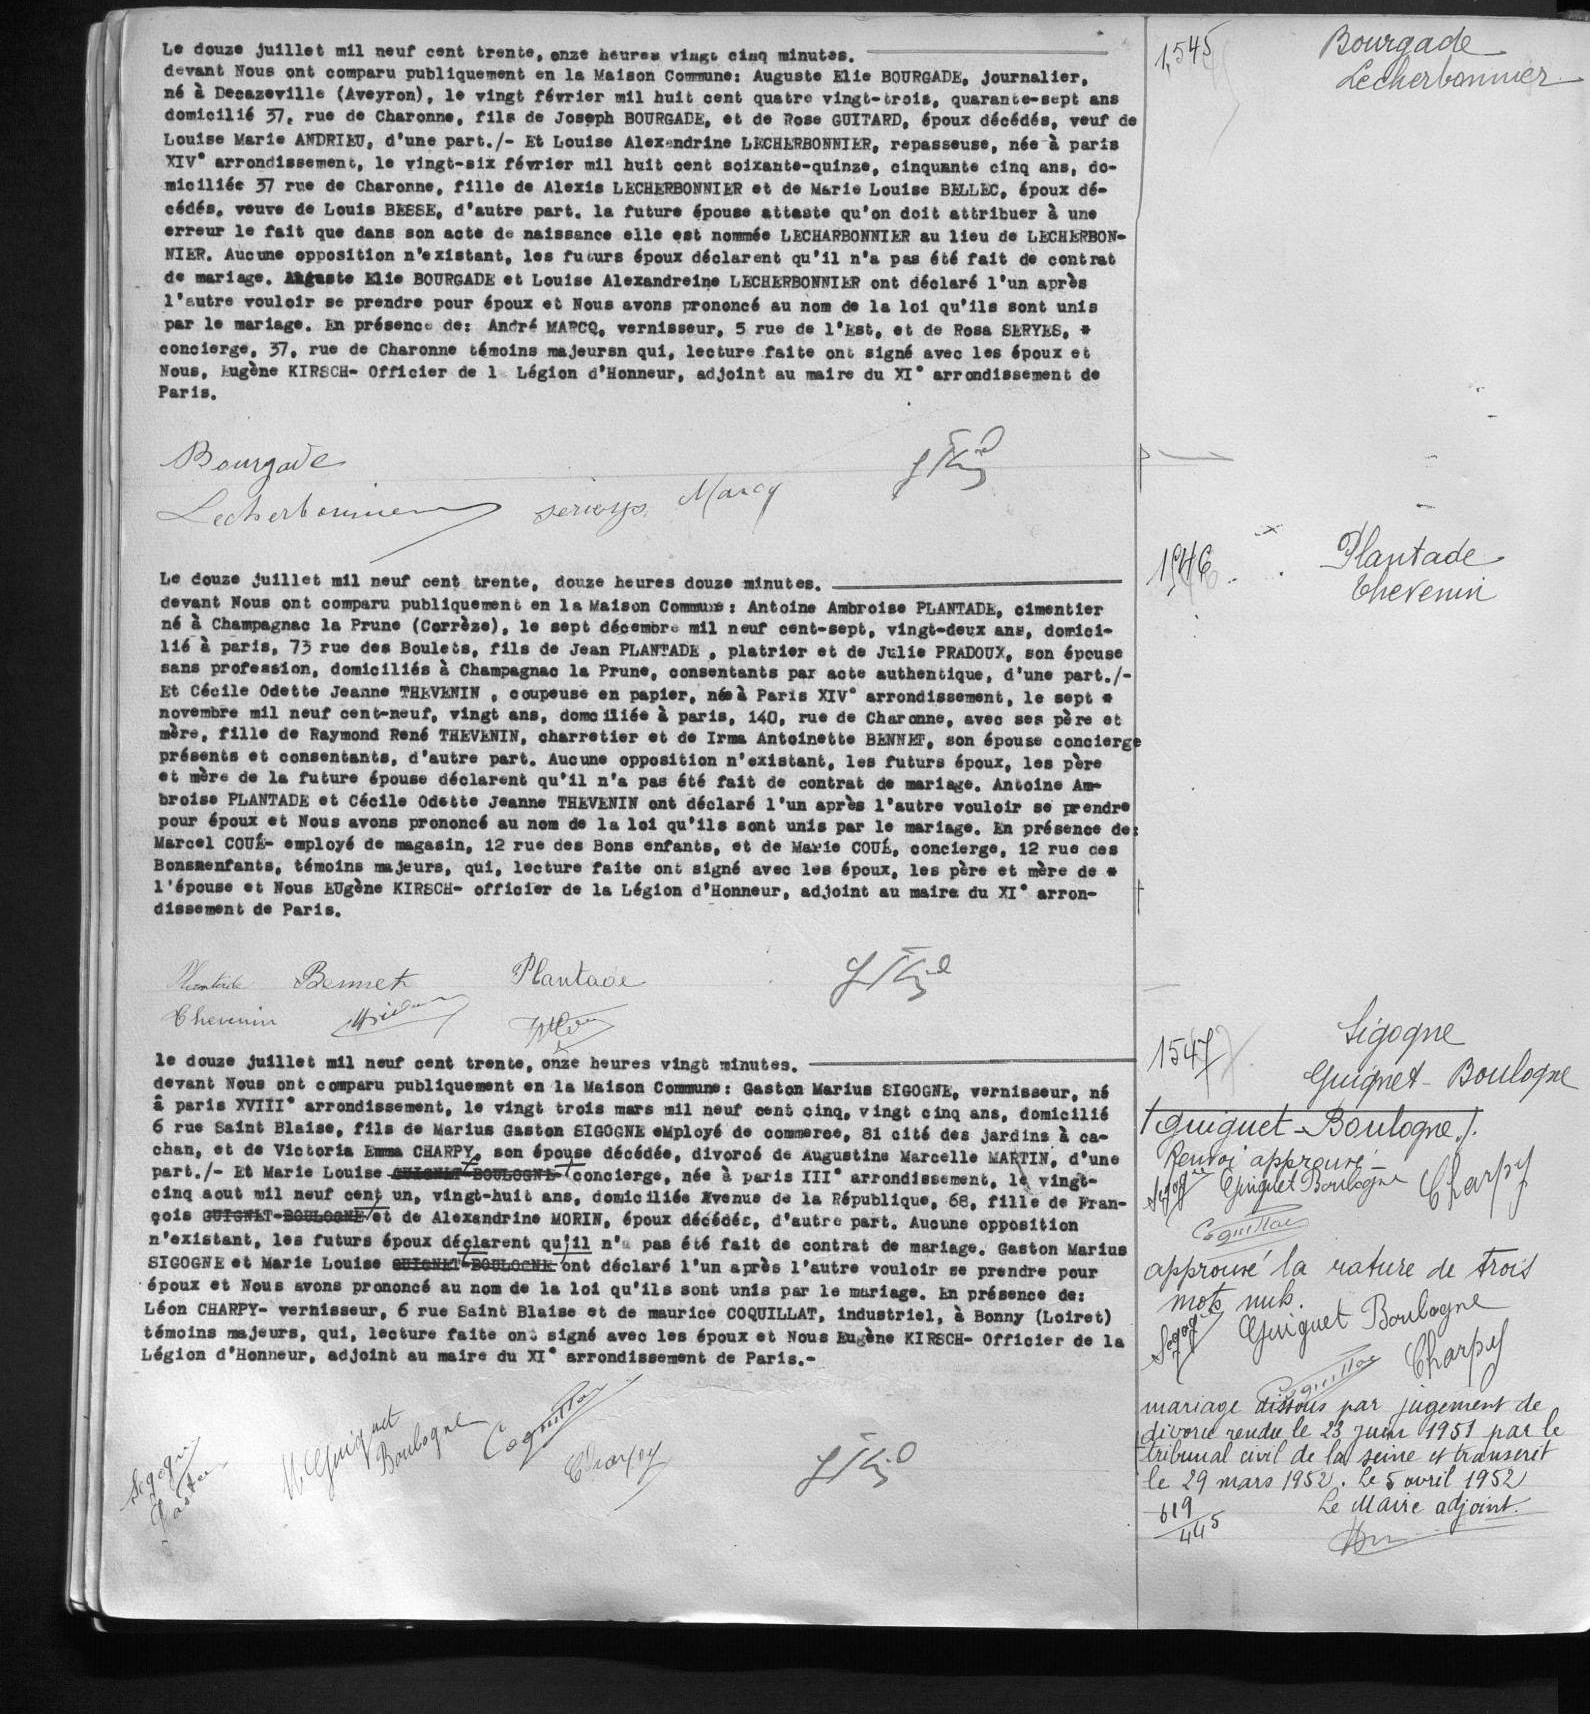Le douze juillet mil neuf cent trente, onze heures vingt minutes ,-
devant Nous ont comparu publiquement en la Maison Commune: Auguste Elie BOURGADE, journalier,
né à Decazeville (Aveyron), le vingt février mil huit cent quatre vingt-trois, quarante-sept ans
domicilié 37, rue de Charonne, fils de Joseph BOURGADE, et de Rose GUITARD, époux décédés, veuf de
Louise Marie ANDRIEU, d'une part ./-Et Louise Alexandrine LECHERBONNIER, repasseuse, née à paris
XIV ° arrondissement, le vingt-six février mil huit cent soixante-quinze, cinquante cinq ans, do-
miciliée 37 rue de Charonne, fille de Alexis LECHERBONNIER et de Marie Louise BELLEC, époux dé-
cédés, veuve de Louis BESSE, d'autre part. La future épouse atteste qu'on doit attribuer à une
erreur le fait que dans son acte de naissance elle est nommé LECHARBONNIER au lieu de LECHERBON-
NIER. Aucune opposition n'existant, les futurs époux déclarent qu'il n'a pas été fait decontrat
de mariage. Auguste Elie BOURGADE et Louise Alexandreine LECHERBONNIER ont déclaré l'un après
l'autre vouloir se prendre pour époux et Nous avons prononcé au nom de la loi qu'ils sont unis
par le mariage. En présence de: André MARCQ, vernisseur, 5 rue de l'Est, et de Rosa SEYRES, *
concierge, 37, rue de Charonne témoins majeursn qui, lecture faite ont signé avec les époux et
Nous, Eugène KIRSCH-Officier de l Légion d'Honneur, adjoint au maire du XI ° arrondissement de
Paris.
Le douze juillet mil neuf cent trente, douze heures douze minutes .-
devant Nous ont comparu publiquement en la Maison Commune: Antoine Ambroise PLANTADE, cimentier
né à Champagnac la Prune (Corrèze), le sept décembre mil neuf cent-sept, vingt-deux ans, domici-
lié à paris, 73 rue des Boulets, fils de Jean PLANTADE, platrier et de Julie PRADOUX, son épouse
sans profession, domiciliés à Champagnac la Prune, consentants par acte authentique, d'une part ./-
Et Cécile Odette Jeanne THEVENIN, coupeuse en papier, née à Paris XIV ° arrondissement, le sept*
novembre mil neuf cent-neuf, vingt ans, domiciliée à paris, 140, rue de Charonne, avec ses père et
mère, fille de Raymond René THEVENI, charretier et de Irma Antoinette BENNET, son épouse concierge
présents et consentants, d'autre part. Aucune opposition n'existant, les futurs époux, les père
et mère de la future épouse déclarent qu'il n'a pas été fait de contrat de mariage. Antoine Am-
broise PLANTADE et Cécile Odette Jeanne THEVENIN ont déclaré l'un après l'autre vouloir se prendre
pour époux et Nous avons prononcé au nom de la loi qu'ils sont unis par le mariage. En présence de:
Marcel COUE-employé de magasin, 12 rue de Bons enfants, et de Marie COUE, concierge, 12 rue ces
Bonsenfants, témoins majeurs, qui, lecture faite ont signé avec les époux, les père et mère de *
l'épouse de Nous EUgène KIRSCH-officier de la Légion d'Honneur, adjoint au maire du XI ° arron-
dissement de Paris.
le douze juillet mil neuf cent trente, onze heures vingt minutes.-
devant Nous ont comparu publiquement en la Maison Commune: Gaston Marius SIGOGNE, vernisseur, né
à paris XVIII ° arrondissement, le vingt trois mars mil neuf cent cinq, vingt cinq ans, domicilié
6 rue Saint Blaise, fils de Marius Gaston SIGOGNE eMployé de commerce, 81 cité des jardins à ca-
chan, et de Victoria Emma CHARPY, son épouse décédée, divorcé de Augustine Marcelle MARTIN, d'un
part ./-Et Marie Louise     Boulogne concierge, née à paris III ° arrondissement, le vingt-
cinq aout mil neuf cent un, vingt-huit ans, domicilié Avenue de la République, 68, fille de Fran-
çois GUIGNET BOULOGNE et de Alexandrine MORIN, époux décédé, d'autre part. Aucune opposition
n'existant, les futurs époux déclarent qu'il n'a pas été fait de contrat de mariage. Gaston Marius
SIGOGNE et Marie Louis GUIGNET BOULOGNE ont déclaré l'un après l'autre vouloir se prendre pour
époux et Nous avons prononcé au nom de la loi qu'ils sont unis par le mariage. En présence de:
Léon CHARPY-vernisseur, 6 rue Saint Blaise et de maurice COQUILLAT, industriel, à Bonny (Loiret)
témoins majeurs, qui, lecture faite ont signé avec les époux et Nous Eugène KIRSCH-Officier de la
Légion d'Honneur, adjoint au maire du XI ° arrondissement de Paris.-
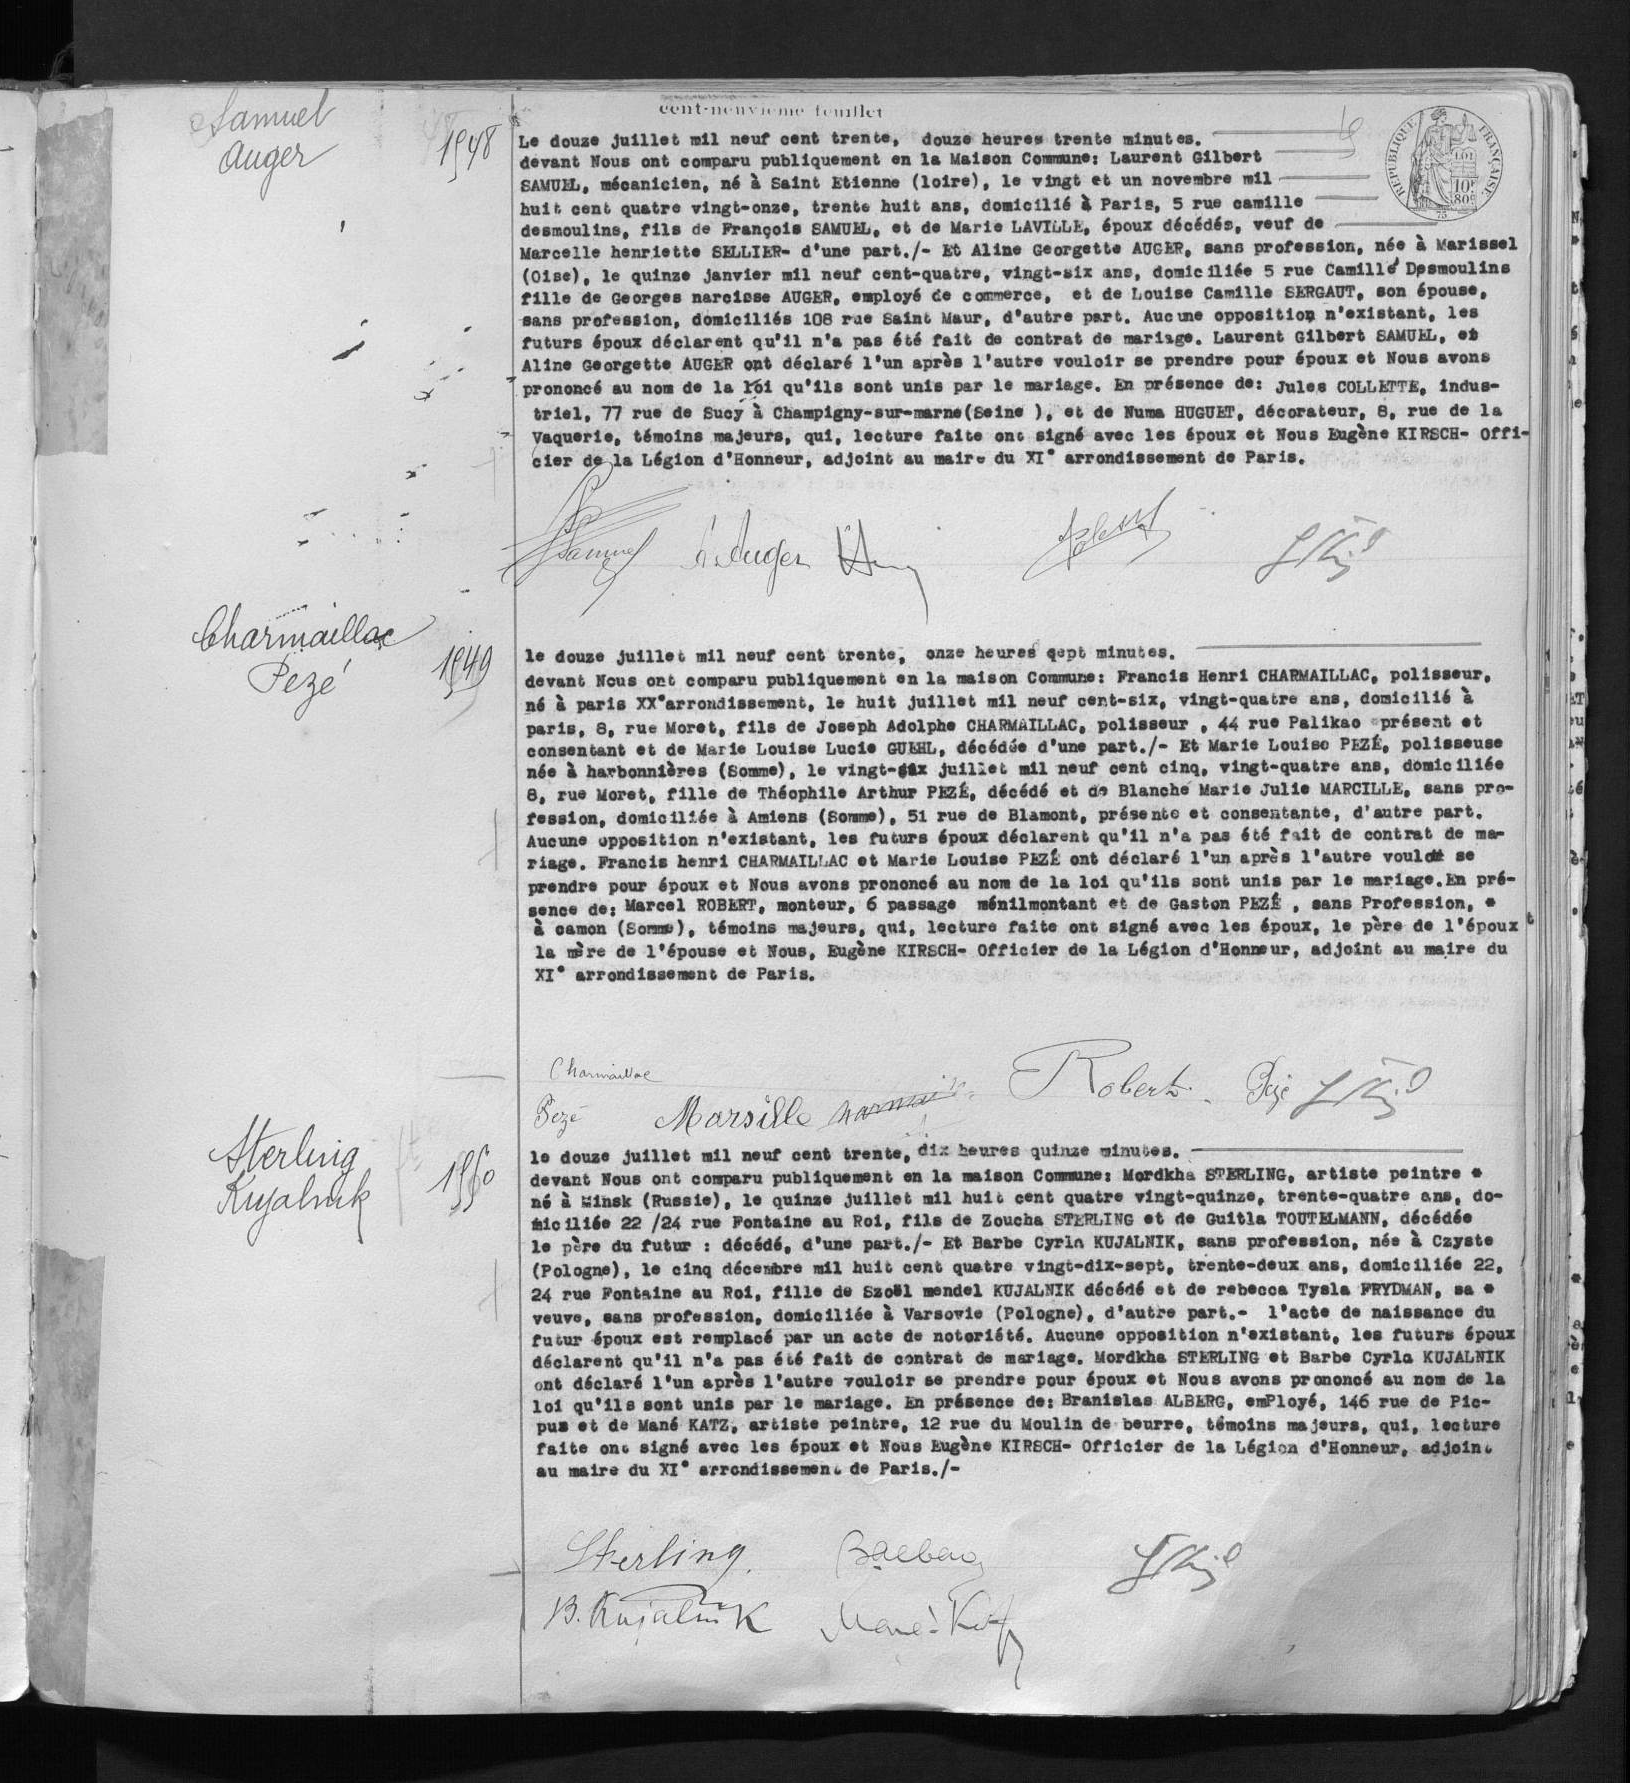Le douze juillet mil neuf cent trente, douze heures trente minutes.-
devant Nous ont comparu publiquement en la Maison Commune: Laurent Gilbert-
SAMUEL, mécanicien, né à Saint Etienne (loire), le vingt et un novembre mil-
huit cent quatre vingt-onze, trente huit ans, domicilié à Paris, 5 rue camille-
des moulins, fils de François SAMUEL, et de Marie LAVILLE, époux décédés, veuf de-
Marcelle henriette SELLIER-d'une part ./-Et Aline Georgette AUGER, sans profession, née à Marissel
(Oise), le quinze janvier mil neuf cent-quatre, vingt-six ans, domiciliée 5 rue Camille Desmoulins
fille de Georges narcisse AUGER, employé de commerce, et de Louise Camille SERGAUT, son épouse,
sans profession, domiciliés 108 rue Saint Maur, d'autre part. Aucune opposition n'existant, les
futurs époux déclarent qu'il n'a pas été fait de contrat de mariage. Laurent Gilbert SAMUEL, et
Aline Georgette AUGER ont déclaré l'un après l'autre vouloir se prendre pour époux et Nous avons
prononcé au nom de la loi qu'ils sont unis par le mariage. En présence de: Jules COLLETTE, indus-
triel, 77 rue du Sucy à Champigny-sur-marne (Seine), et de Numa HUGUET, décorateur, 8, rue de la
Vaquerie, témoins majeurs, qui, lecture faite ont signé avec les époux et Nous Eugène KIRSCH-Offi
cier de la Légion d'Honneur, adjoint au maire du XI ° arrondissement de Paris.
le douze juillet mil neuf cent trente, onze heures qept minutes.-
devant Nous ont comparu publiquement en la maison Commune: Francis Henri CHARMAILLAC, polisseur,
né à paris XX ° arrondissement, le huit juillet mil neuf cent-six, vingt-quatre ans, domicilié à
paris, 8, rue Moret, fils de Joseph Adolphe CHARMAILLAC, polisseur, 44 rue Palikao présent et
consentant et de Marie Louise Lucie GUEUL, décédée d'une part ./-Et Marie Louise PEZE, polisseuse
née à harbonnières (Somme), le vingt-six juillet mil neuf cent cinq, vingt-quatre ans, domiciliée
8, rue Moret, fille de Théophile Arthur PEZE, décédé et de Blanche Marie Julie MARCILLE, sans pro-
fession, domiciliée à Amiens (Somme), 51 rue de Blamont, présente et consentante, d'autre part.
Aucune opposition n'existant, les futurs époux déclarent qu'il n'a pas été fait de contrat de ma-
riage. Francis henri CHARMAILLAC et Marie Louise PEZE ont déclaré l'un après l'autre vouloir de
prendre pour époux et Nous avons prononcé au nom de la loi qu'ils sont unis par le mariage. En pré-
sence de: Marcel ROBERT, monteur, 6 passage ménilmontant et de Gaston PEZE, sans Profession, *
à camon (Somme), témoins majeurs, qui, lecture faite ont signé avec les époux, le père de l'époux
la mère de l'épouse et Nous, Eugène KIRSCH-Officier de la Légion d'Honneur, adjoint au maire du
XI ° arrondissement de Paris.
le douze juillet mil neuf cent trente, dix heures quinze minutes. -
devant Nous ont comparu publiquement en la maison Commune: Mordkha STERLING, artiste peintre *
né à Minsk (Russie), le quinze juillet mil huit cent quatre vingt-quinze, trente-quatre ans, do-
miciliée 22/ 24 rue Fontaine au Roi, fils de Zoucha STERLING et de Guilta TOUTELMANN, décédée
le père du future: décédé, d'une part ./-Et Barbe Cyrin KUJALNIK, sans profession, née à Czyste
(Pologne), le cinq décembre mil huit cent quatre vingt-dix-sept, trente-deux ans, domiciliée 22,
24 rue Fontaine au Roi, fille de Szoël mendel KUJALNIK décédé et de rebecca Tysla FRYDMAN, sa *
veuve, sans profession, domiciliée à Varsovie (Pologne), d'autre part .-l'acte de naissance du
futur époux est remplacé par un acte de notoriété. Aucune opposition n'existant, les futurs époux
déclarent qu'il n'a pas été fait de contrat de mariage. Mordkha STERLING et Barbe Cyrlo KUJALNIK
ont déclaré l'un après l'autre vouloir se prendre pour époux et Nous avons prononcé au nom de la
loi qu'ils sont unis par le mariage. En présence de: Branisla ALBERG, emPloyé, 146 rue de la Pic-
pus et de Mané KATZ, artiste peintre, 12 rue du Moulin de beurre, témoins majeurs, qui, lecture
faite ont signé avec les époux et Nous Eugène KIRSCH-Officier de la Légion d'Honneur, adjoint
au maire du XI ° arrondissement de Paris ./-